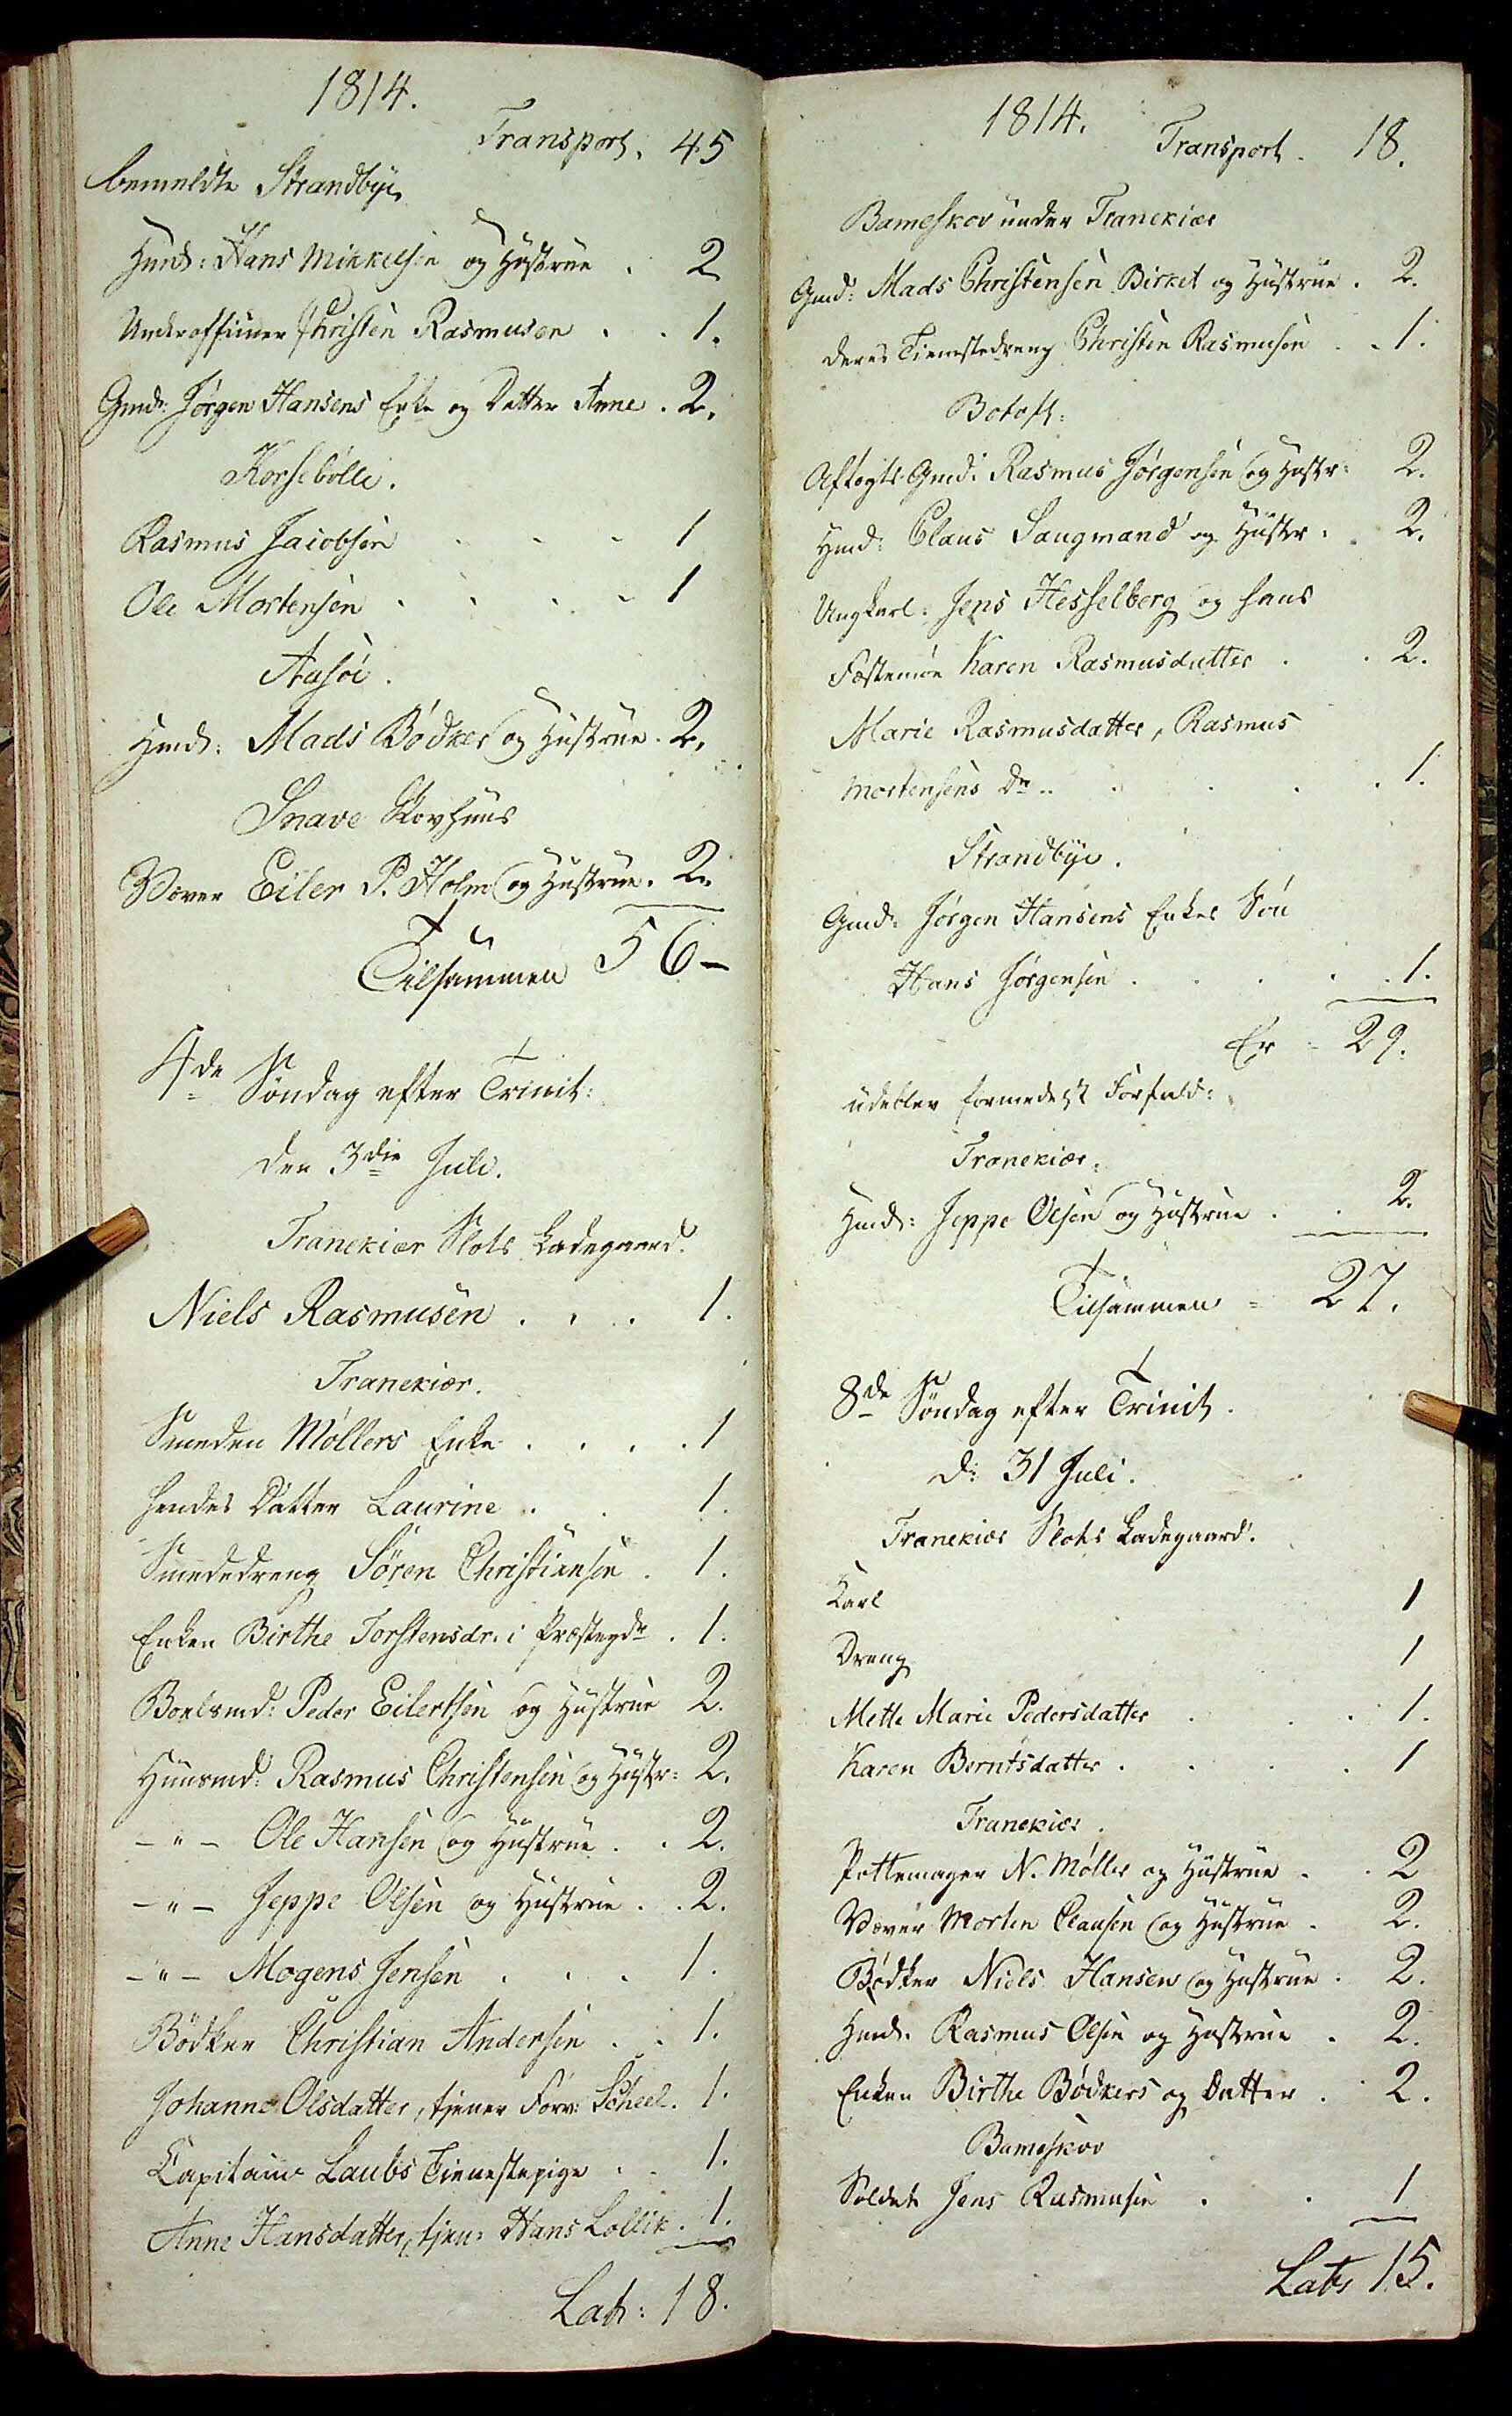1814.
Transport . 45
bemeldte Strandbye.
Hmd: Hans Mikkelsen og Hustrue . 2
Underofficeer Christen Rasmusen . . 1.
Gmd: Jørgen Hansens Enke og Datter Anne .2.
Korsebølle.
Rasmus Jacobsen . . . 1
Ole Mortensen . . . . 1
Aasør
Hmd. Mads Bødker og Hustrue . 2.
Snave Skovhuus
Væver Eiler P. Holm og Hustrue . 2.
Tilsammen 56
4de Søndag efter Trinit:
den 3die Juli.
Tranekiær Slots Ladegaard.
Niels Rasmusen . . . 1.
Tranekiær.
Smeden Møllers Enke . . . . 1
hendes Datter Laurine . . 1.
Smededreng Søren Christiansen . 1.
Enken Birthe Torstensdr. i Præstegdr . 1.
Boelsmd: Peder Eilertsen og Hustrue 2.
Huusmd: Rasmus Christensen og Hustr: 2.
-"- Ole Hansen og Hustrue . . 2.
-"- Jeppe Olsen og Hustrue . . 2.
-"- Mogens Jensen . . . 1.
Bødker Christian Andersen . . 1.
Johanne Olsdatter, tjener Forv: Scheel . 1.
Capitain Laubs Tienestepige . . 1.
Anne Hansdatter, tjen: Hans Lollik . 1.
Lat: 18.
1814.
Transport. 18.
Bameskov under Tranekiær
Gmd: Mads Christensen Birket og Hustrue . 2.
deres Tienestedreng Christen Rasmusen . . 1.
Botoft:
Aftægts Gmd. Rasmus Jørgensen og Hustr: 2.
Hmd: Claus Saugmand og Hustr: . 2.
Ungkarl: Jens Hesselberg og hans
Fæstemøe Karen Rasmusdatter . . 2.
Marie Rasmusdatter, Rasmus
Mortensens Dr . . . . . . 1.
Strandbye.
Gmd: Jørgen Hansens Enkes Søn
Hans Jørgensen . . . . . 1.
Er: 29.
udeblev formedelst Forfald:
Tranekiær
Hmd: Jeppe Olsen og Hustrue . . 2.
Tilsammen= 27.
8de Søndag efter Trinit
d: 31 Juli.
Tranekiær Slota Ladegaard.
Karl 1
Dreng 1
Mette Marie Pedersdatter . . . . 1.
Karen Berntsdatter . . . . 1
Tranekiær
Pottemager N. Møller og Hustrue . . 2
Væver Morten Clausen og Hustrue . 2.
Bødker Niels Hansens og Hustrue . 2.
Hmd. Rasmus Olsen og Hustrue . 2.
Enken Birthe Bøfkers og Datter . 2.
Bameskov
Soldat Jens Rasmusen . . 1
Lat 15.
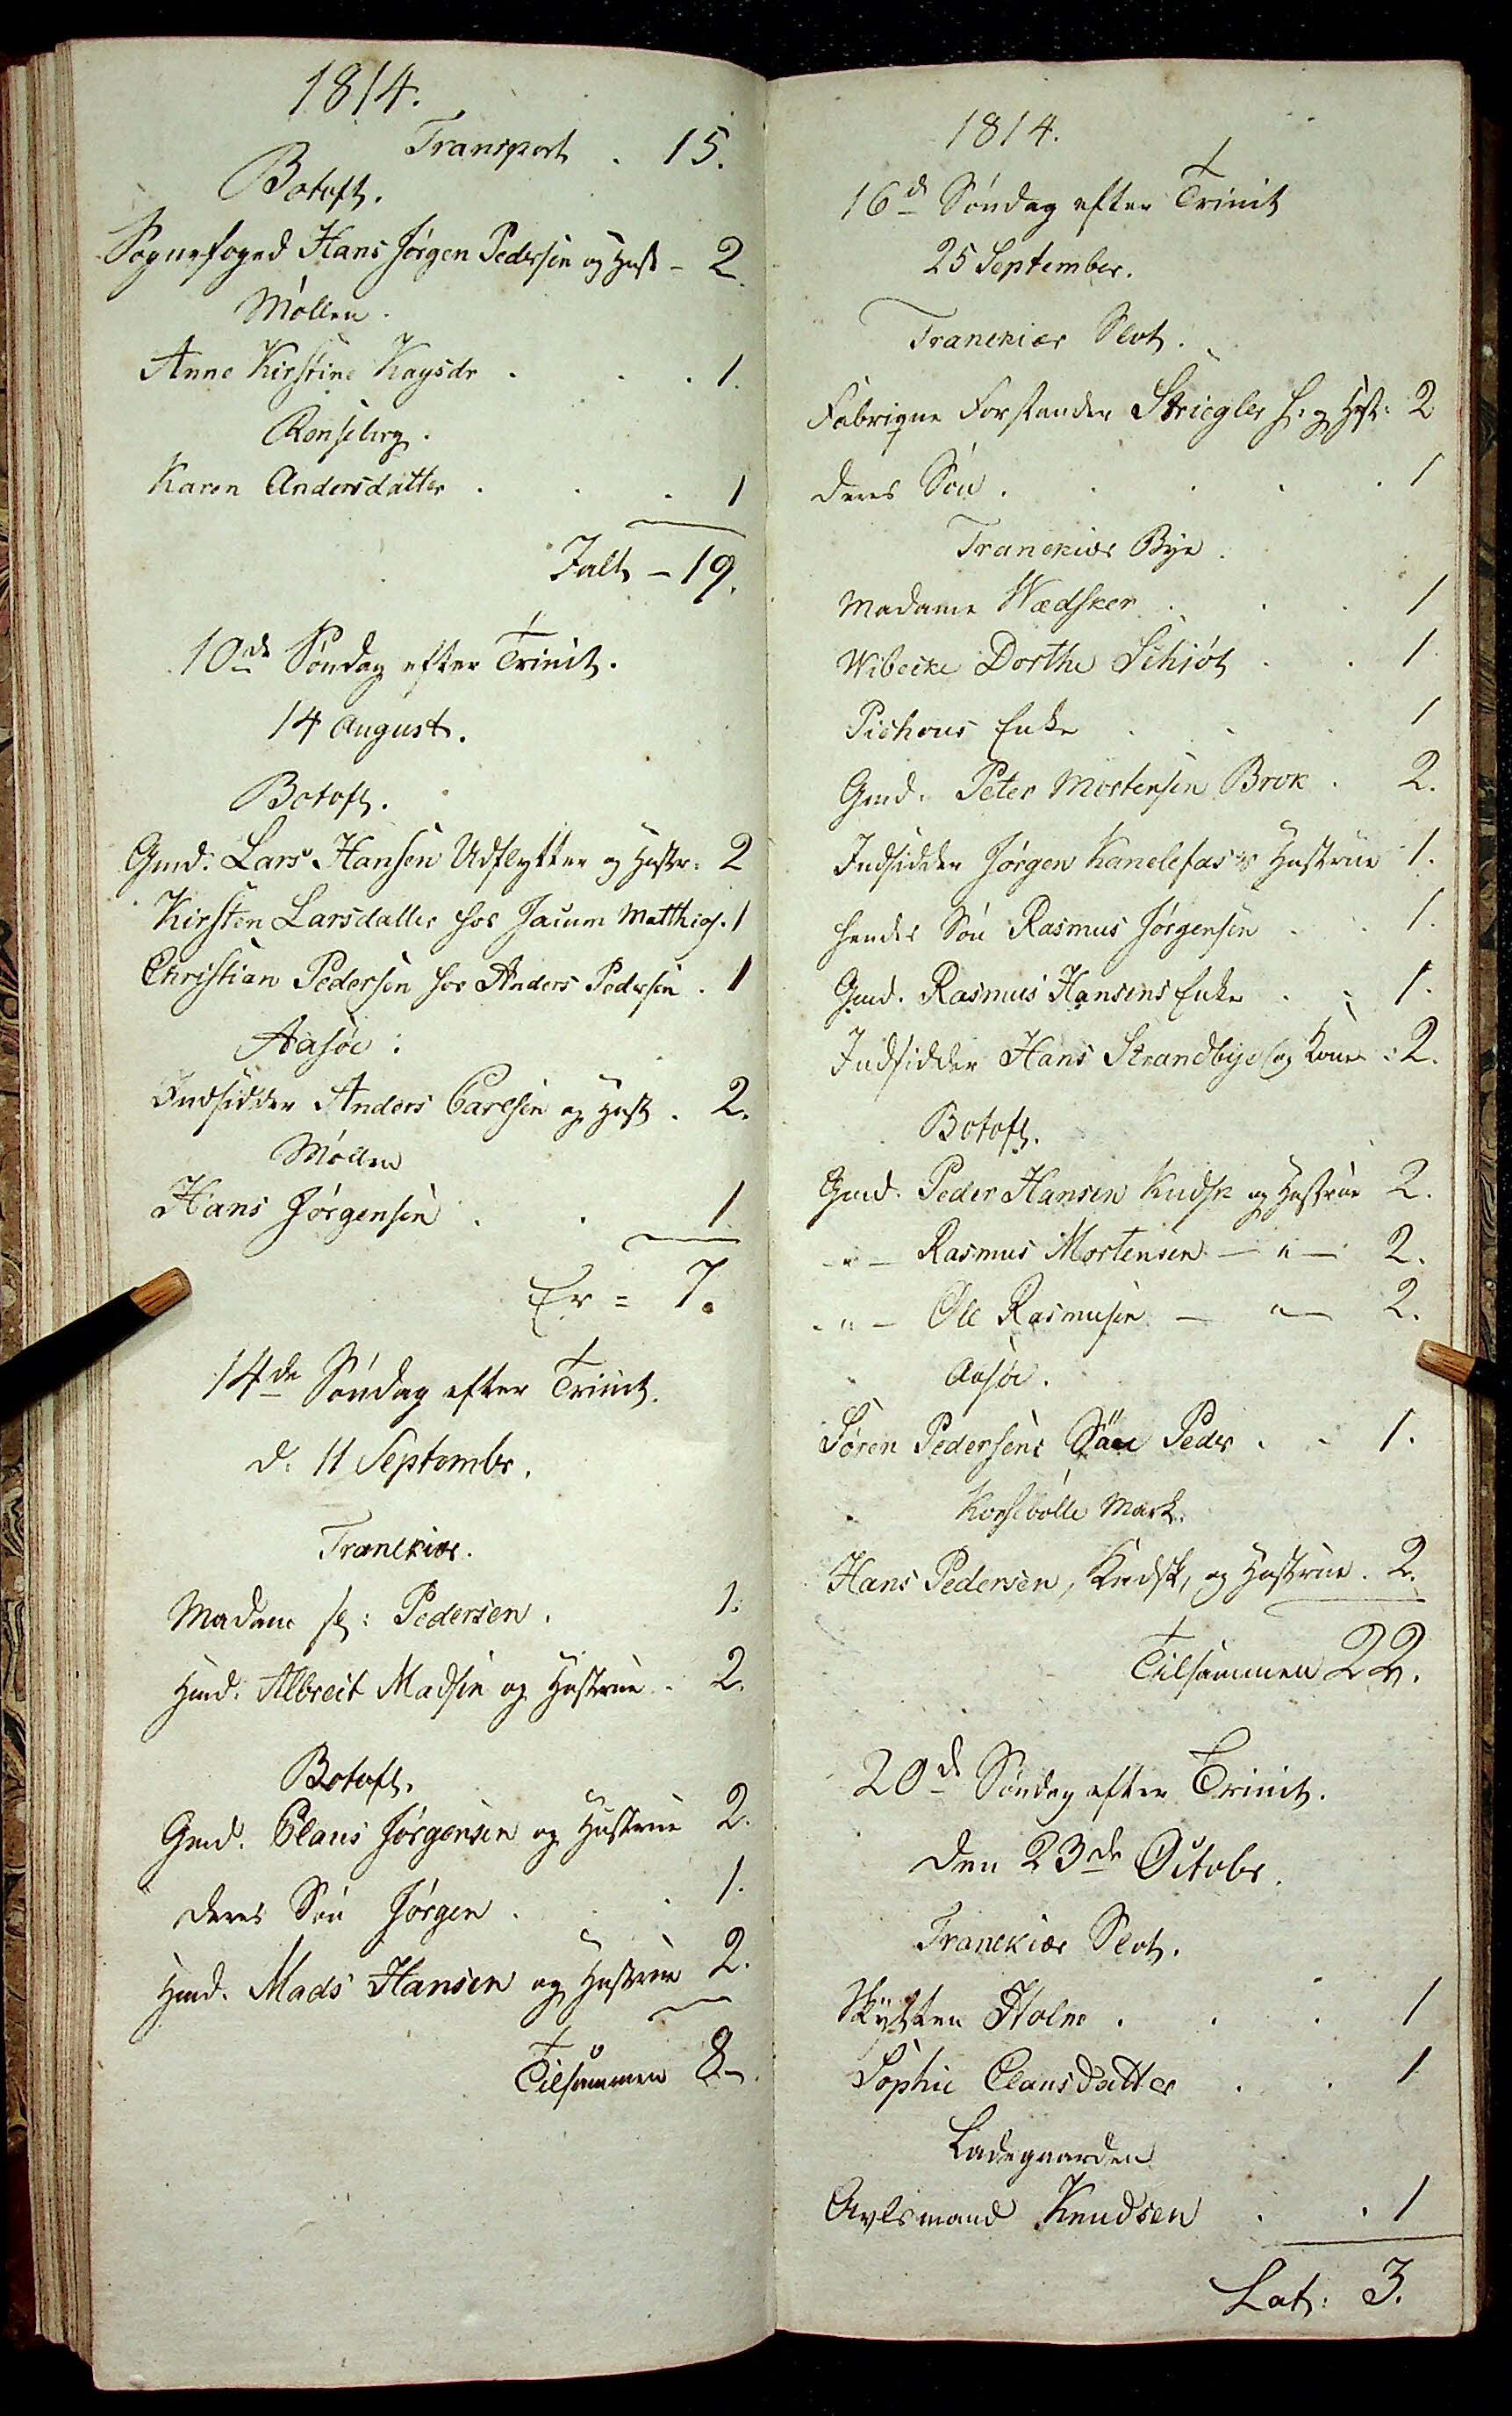1814.
Transport . 15.
Botoft.
Sognefoged Hans Jørgen Pedersen og Hust - 2
Møllen.
Anne Kirstine Kaysdr . . . 1.
Renseberg
Karen Andersdatter . . . 1
Ialt - 19.
10de Søndag efter Trinit.
14 August.
Botoft.
Gmd: Lars Hansen Udflytter og Hustr: 2
Kirsten Larsdatter hos Jocum Matthias . 1
Christian Pedersen hos Anders Pedersen . 1
Aasøe.
Indsidder Anders Carlsen og Hust . 2.
Møllen
Hans Jørgensen . . 1
Er= 7.
14de Søndag efter Trinit.
d: 11 Septembr.
Tranekiær.
Madam sl: Pedersen . 1.
Hmd: Albrect Madsen og Hustrue . 2.
Botoft
Gmd. Claus Jørgensen og Hustrue 2.
ders Søn Jørgen . . . 1.
Hmd. Mads Hansen og Hustrue 2.
Tilsammen 8-
1814.
16de Søndag efter Trinit
25 September.
Tranekiær Slot.
Fabrique Forstander Striegler ##: Hstr: 2
dere Søn . . . . . 1
Tranekiær Bye.
Madame Wædsker . . . 1
Wibecke Dorthe Schiøt . . 1
Pichous Enke . . . 1
Gmd: Peter Mortensens Brok . 2.
Indsidder Jørgen Kanelefas og Hustrue 1.
hendes Søn Rasmus Jørgensen . . 1.
Gmd. Rasmus Hansens Enke . . 1
Indsidder Hans Strandbye og Kone . 2.
Botoft.
Gmd. Peder Hansen Kudsk og Hustrue 2.
-"- Rasmus Mortensen  -"- 2.
-"- Ole Rasmussen -"- 2.
Aasøe.
Søren Pedersens Søn Peder . . 1.
Korsebølle Mark.
Hans Pedersen, Kudsk, og Hustrue . 2.
Tilsammen 22.
20de Søndag efter Trinit.
den 23de Octobr:
Tranekiær Slot.
Skytten Holm . . . 1
Sophie Clausdatter . . 1
Ladegaarden
Avlsmand Knudsen . . 1
Lat: 3.